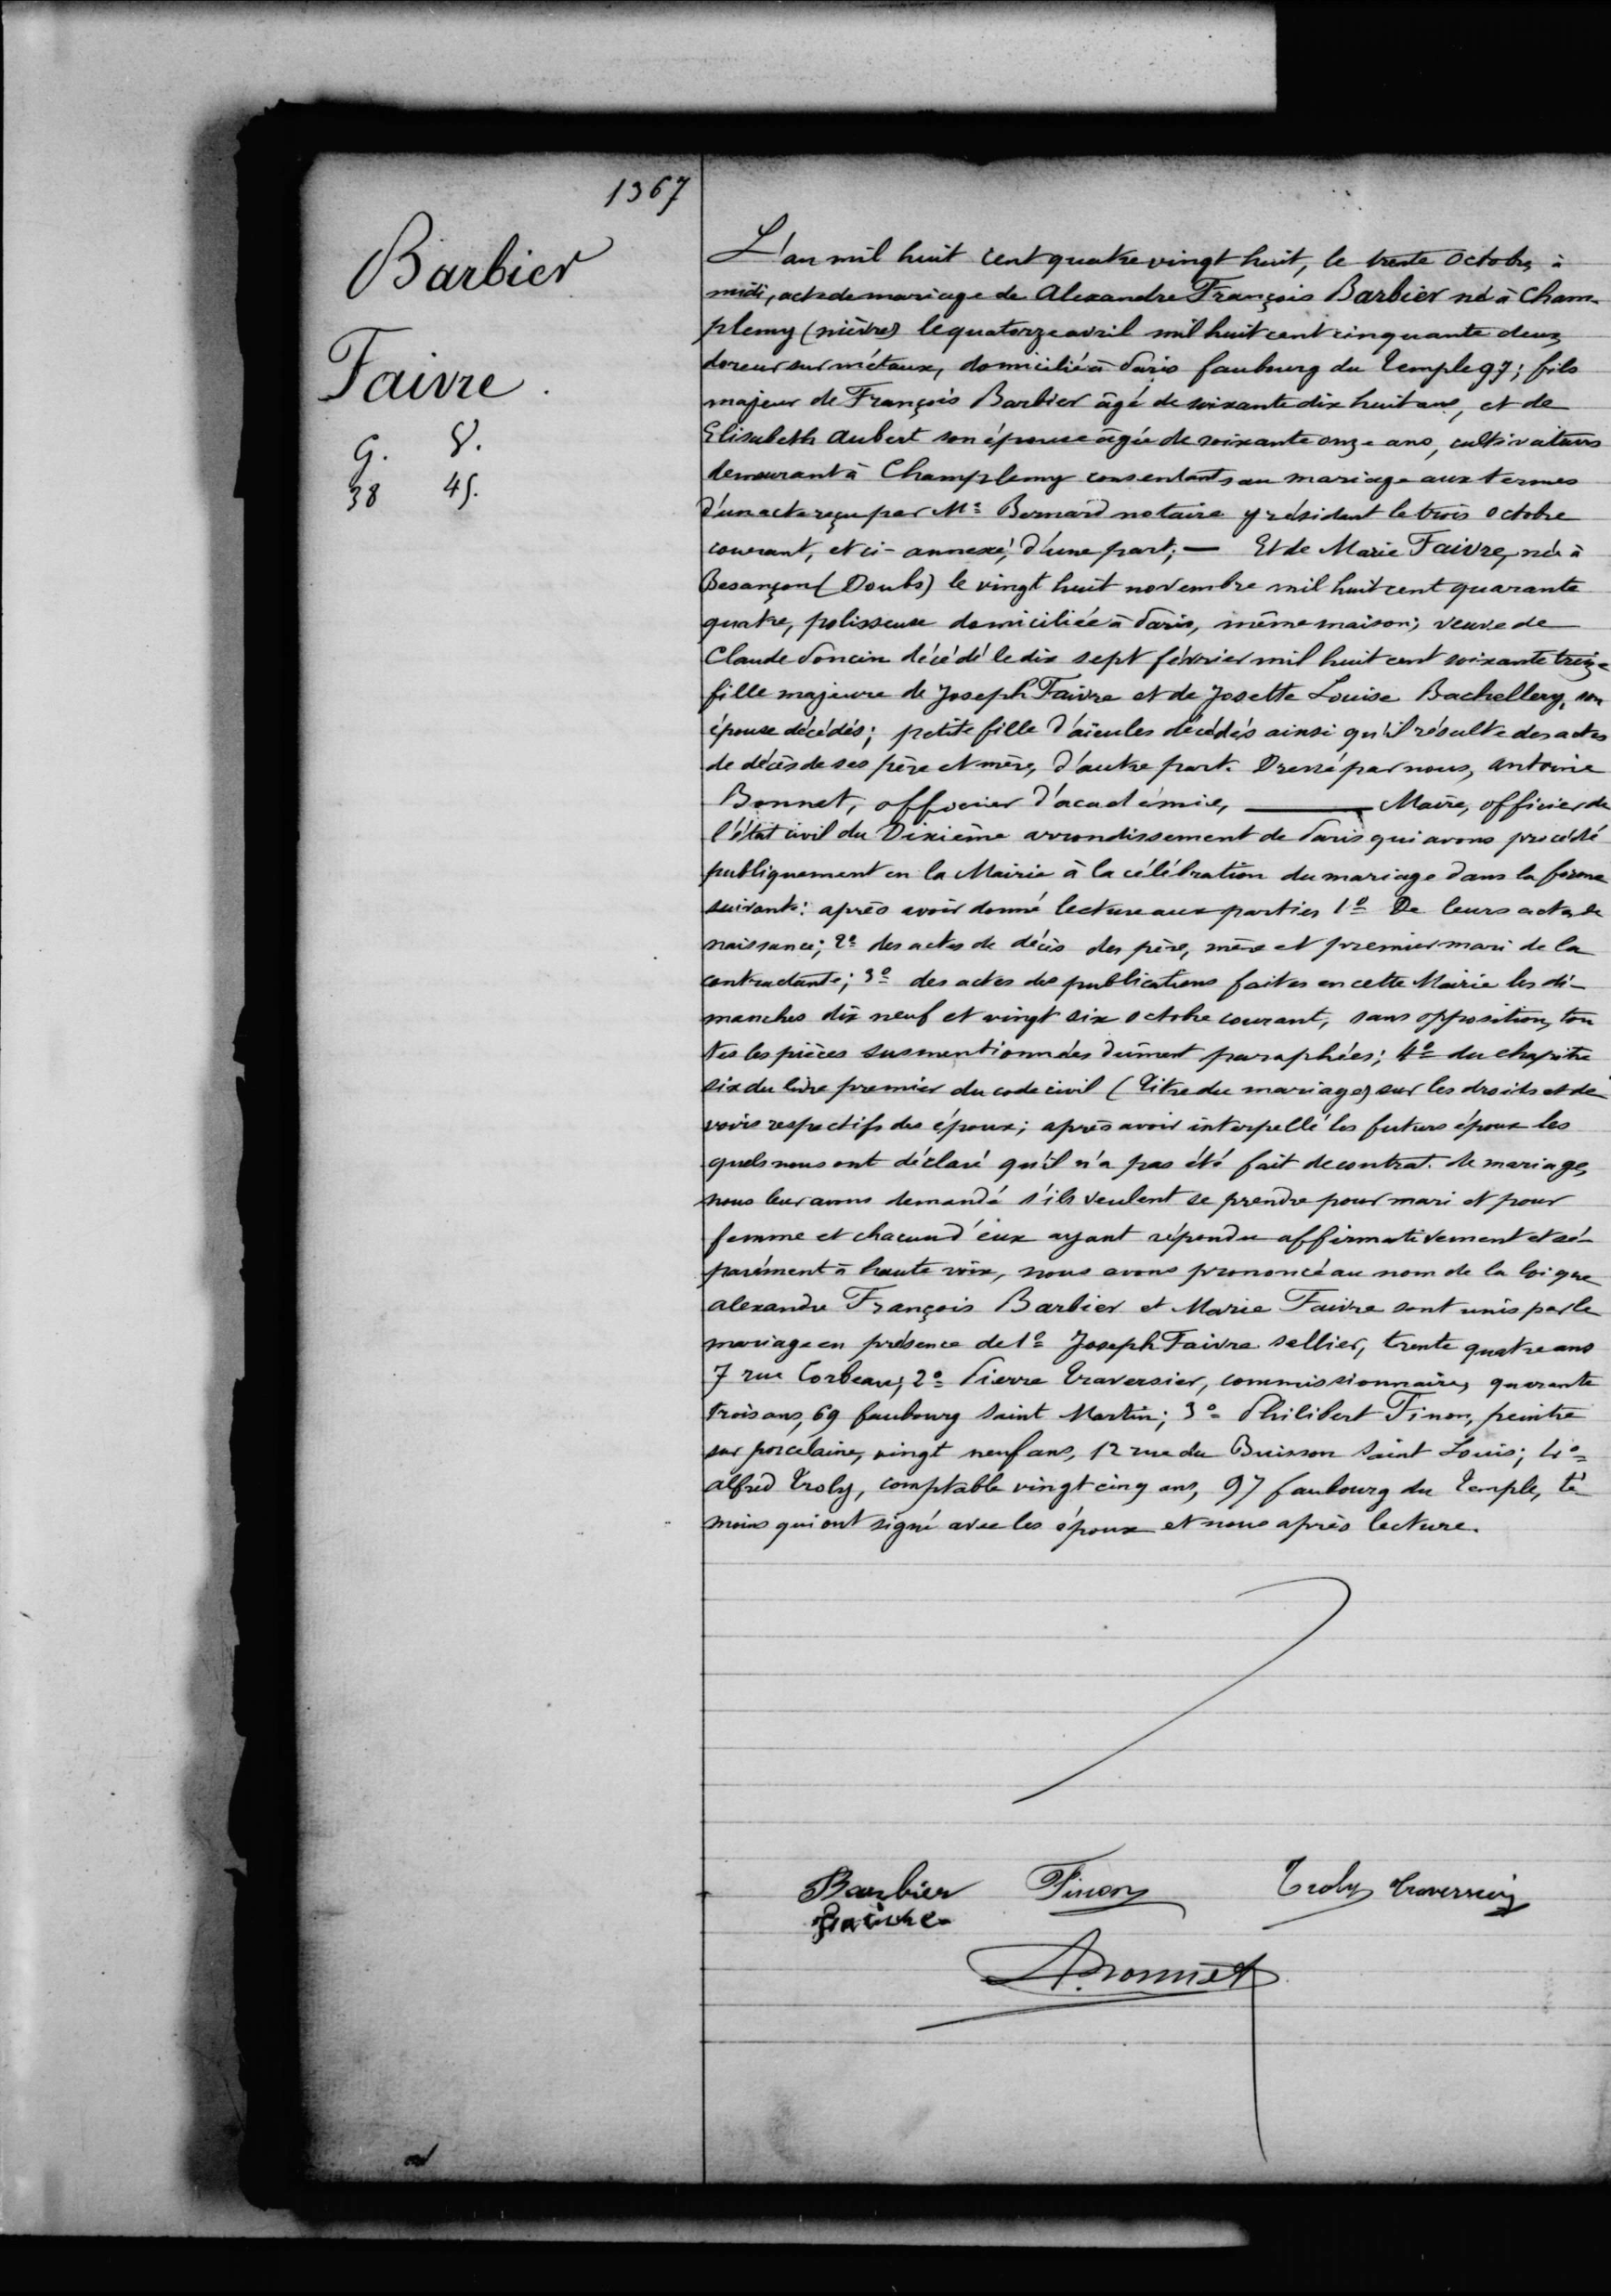1967
Barbier
Faivre
G. V.
38 45
L'an mil huit cent quatre vingt huit, le trente octobre à
midi, actedemariage de Alexandre François Barbier né à Cham-
plemy (Nièvres) le quatorze avril mil huit cent cinquante deux
doreur sur méteaux, domiciliés à Paris faubourg du Temple 97; fils
majeur de François Bartier âgé de soixante dix huit ans, et de
Elisabeth Aubert son épouse âgée de soixante onze ans, cultivateurs
demeurant à Complemy consentants au mariage aux terme
d'un acte reçu par M° Bosnard notaire préseidant le trois octobre
courant, et ci-annexé, d'une part; -Et de Marie Faivre, nè à
Besançon (Doubs) le vingt huit novembre mil huit cent quarante
quatre, polisseuse domiciliée à Paris, même maison; veuve de
Claude Soncine décédé le dix sept février mil huit cent soixante treize
fille majeure de Joseph Faivre et de Josette Louise Bachellery, son
épouse décédés; petite fille d'aïeules décédés ainsi qu'il résulte des actes
de décès de ses père et mère, d'autre part. Dressé par nous, antoine
Bonnet, officier d'académie, -Maire, officier de
l'état civil du Dixième arrondissement de Paris qui avons procédé
publiquement en la Mairie à la célébration du mariage dans la forme
suivante: après avoir donné lecture aux parties 1° De leurs actes de
naissance; 2° des actes de décès du père, mère et premier mari de la
contractante; 3° des actes de publications faites en cette Mairie les di-
manches dix neuf et vingt six octobre courant, sans opposition, tou
tes les pièces susmentionnées dûment paraphées; 4° du chapitre
six du livre premier du code civil (Titre du mariage) sur les droits et de-
voirs respectifs des époux; après avoir interpellé les futurs époux les
quels nout ont déclaré qu'il n'a pas été fait de contrat de mariage,
nous leur avons demandé s'ils veulent se prendre pour mari et pour
femme et chacun d'eux ayant répondu affirmativement et sé-
parèment à haute voix, nous avons prononcé au nom de la loi que
alexandre François Barbier et Marie Faivre son unis par le
mariage en présence de 1° Joseph Faivre Sellier, trente quatre ans
7 rue Corbeau; 2° Fierre Traversier, commissionnaire, quarante
trois ans, 69 faubourg Saint Martin; 3° Philibert Finon, peintre
sur porcelain, vingt neuf ans, 12 rue du Buisson Saint Louis; 4°
Alfre Troly, comptable vingt cinq ans, 97 faubourg de Temple, té-
moins qui ont signé avec les époux et nous après lecture.
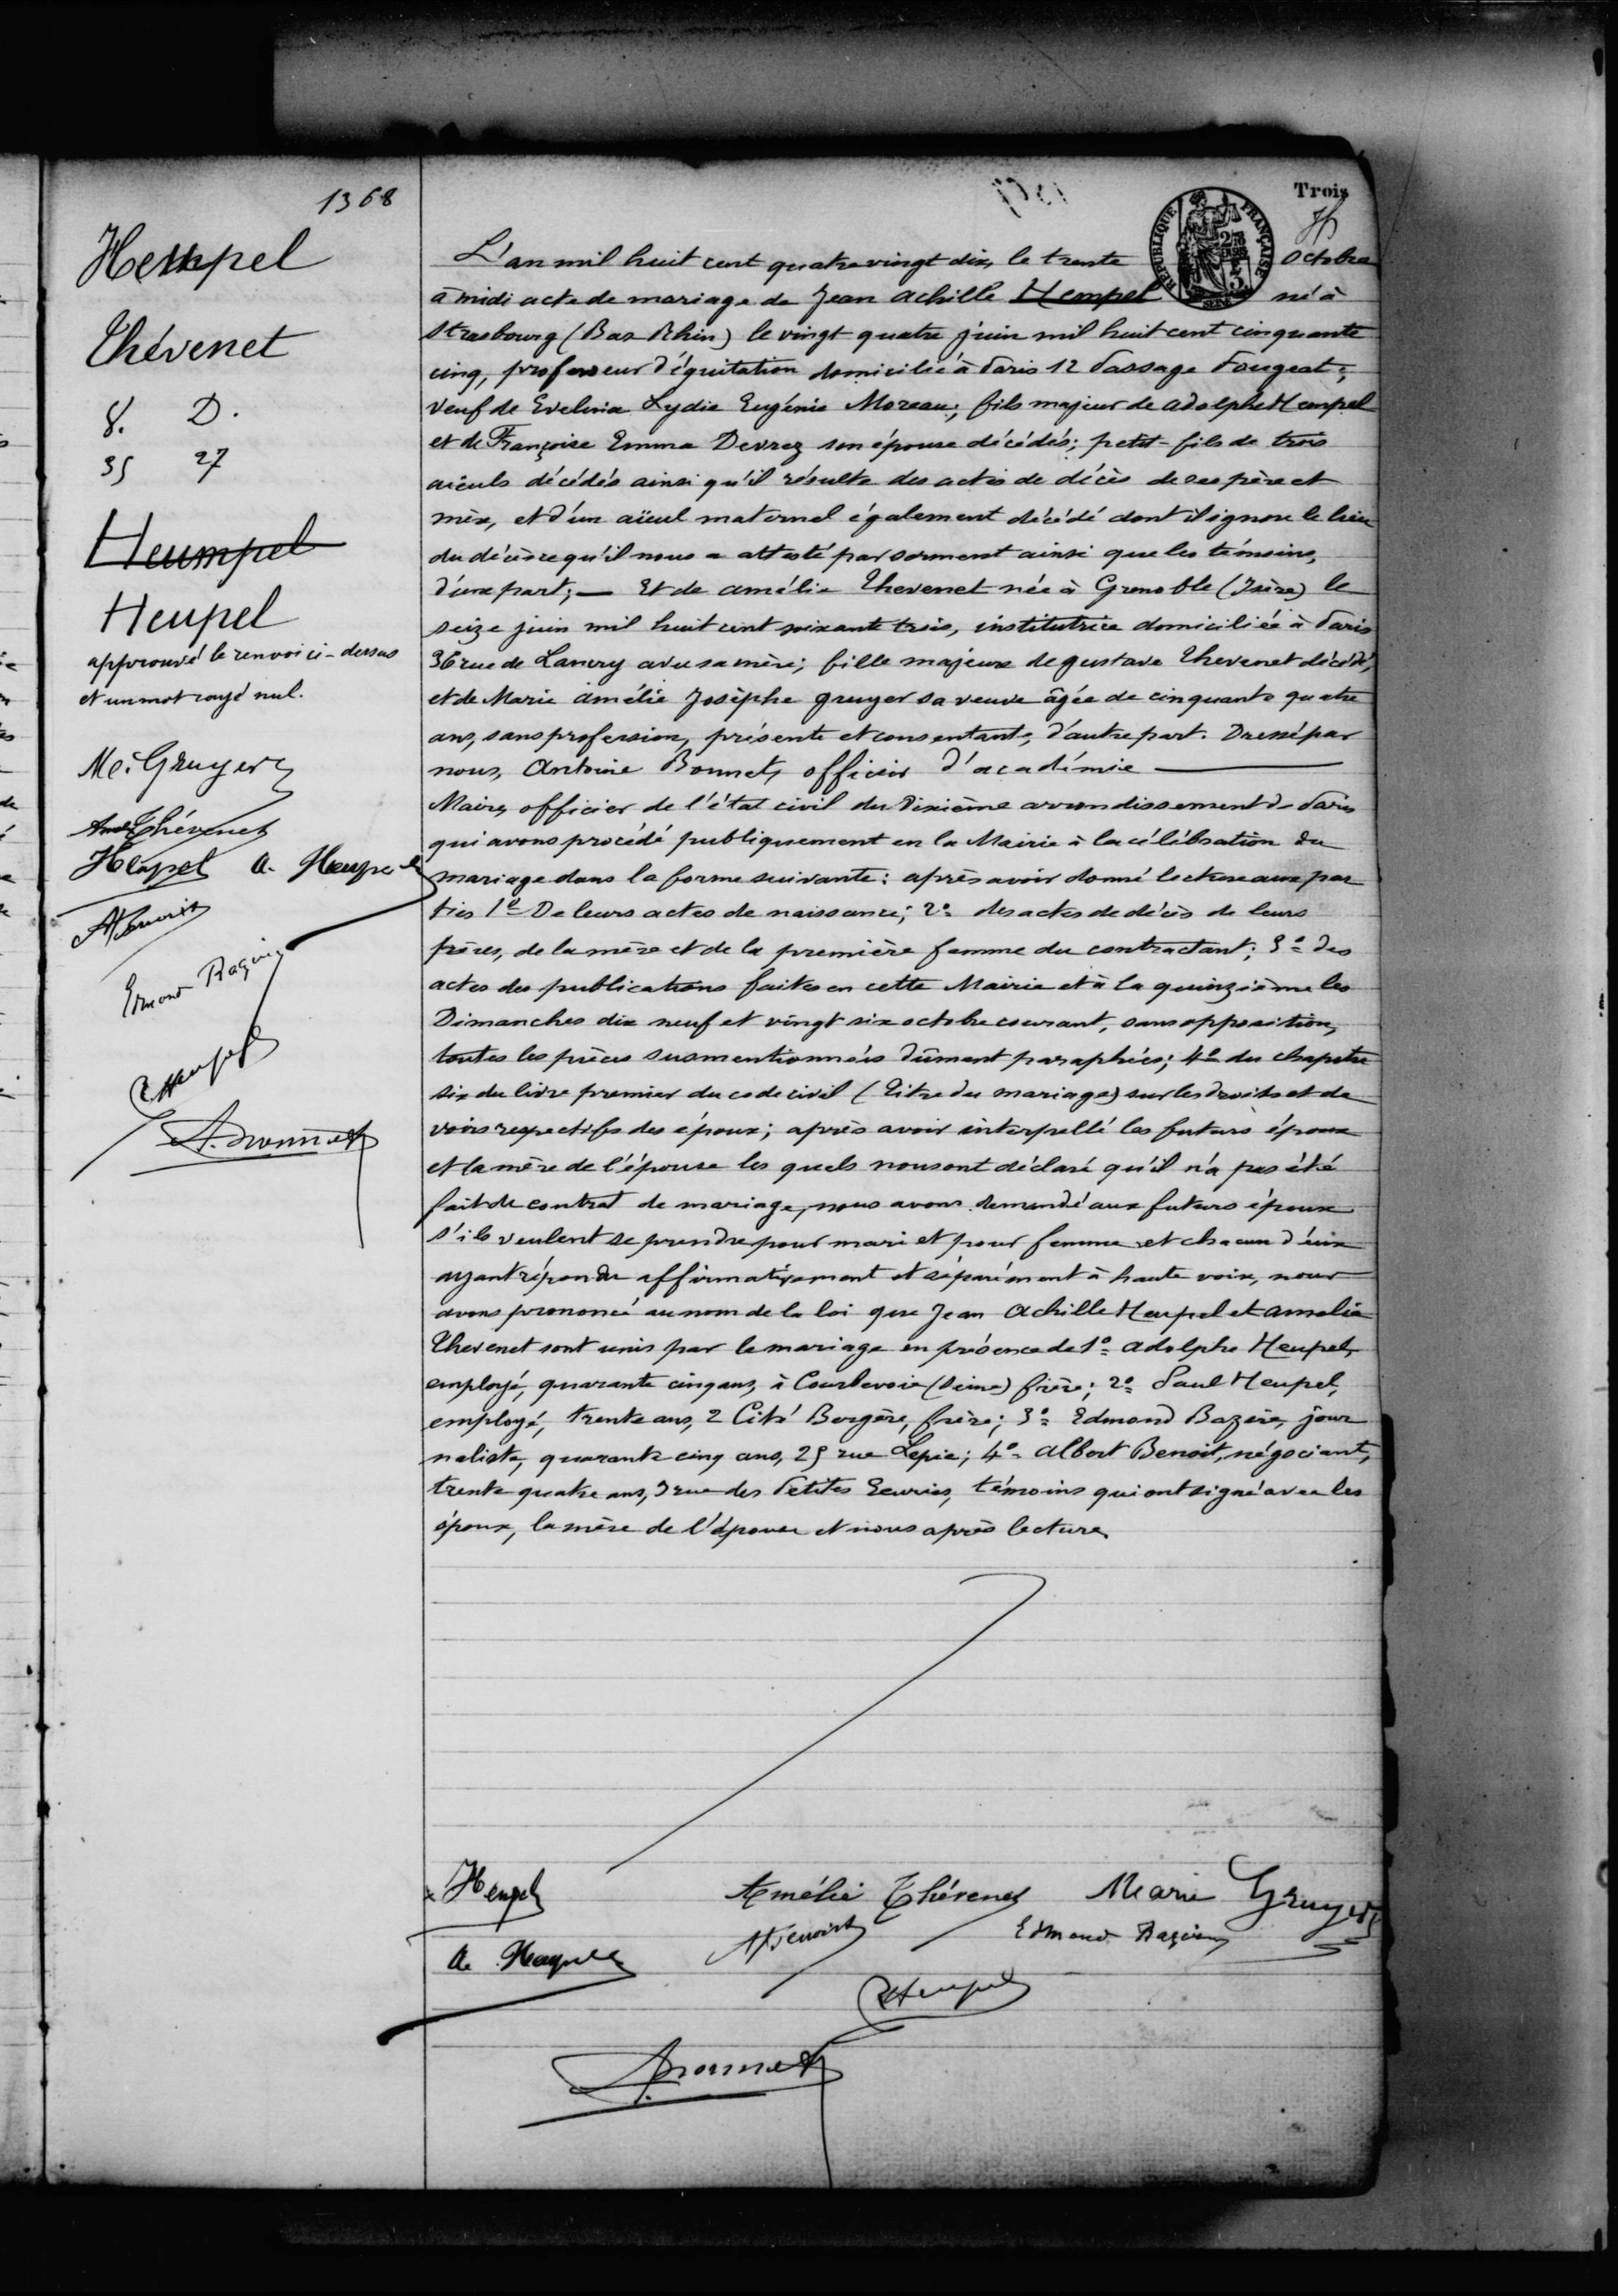1368
Hesspel
Chévenet
L'an mil huit cent quatre vingt dix, le trente octobre
à midi acte de mariage de Jean Achille Hempel né à
Strasbourg (Bas Rhin) le vingt quatre juin mil huit cent cinquante
cinq, professeur d'équitation domicilié à Paris 12 Passage Fougeat;
Veuf de Evelinia Lydia Eugénie Mozeau; fils majeur de adolphe Hempel
et de Françoise Emma Devrez son épouse décédés; petit-fils de trois
aieuls décédés ainsi qu'il résulte des actes de décès de ses père et
mère, et d'un aïeul maternel également décédé dont il ignore le lieu
du décès ce qu'il nous a attesté par serment ainsi que les témoins,
d'une part; -Et de amélia Thevenet née à Grenoble (Isère) le
seize juin mil huit cent soixante trois, institutrice domiciliée à Paris
96 rue de Lancry avec sa mère; fille majeure de Gustave Thevenet décédé,
et de Marie amélie Josèphe Gruyer sa veuve âgée de cinquante quatre
ans, sans profession, présent et consentant; d'autre part. Dressé par
nous, Antoine Bonnet, officier d'académie-
Maire, officier de l'état civil du dixième arrondissement, Paris
qui avons procédé publiquement en la Mairie à la célébration du
mariage, dans la forme suivante: après avoir donné lecture aux par
ties 1° De leurs actes de naissance; 2° des actes de décès de leurs
pères, de la mère et de la première femme du contractant; 3° des
actes des publications faites en cette Mairie et à la quinzième les
Dimanches dix neuf et vingt six octobre courant, sans opposition,
toutes les pièces susmentionnées dûment paraphées; 4° du chapitre
six du livre premier du code civil (Titre du mariage) sur les droits et de
voirs respectifs des époux; après avoir interpellé les futurs époux
et la mère de l'épouse les quels nous ont déclaré qu'il n'a pas été
fait de contrat de mariage, nous avons demandé aux futurs époux
s'ils veulent se prendre pour mari et pour femme et chacun d'eux
ayant répondu affirmativement et séparèment à haute voix, nous
avons prononcé au nom de la loi que Jean Achille Hempel et amelia
Chevenet sont unis par le mariage en présence de 1° Adolphe Henpel,
employé, quarante cinq ans, à Courbevoie (Seine) frère; 2° Paul Henpel
employé; trente ans, 2 Cité Bergère, frère; 3° Edmond Bazère jour
naliste, quarante-cinq ans, 25 rue Lepic; 4° Albert Benoit, négociant,
trente quatre ans, 9 rue des Petites Leuriers, témoins qui ont signé avec les
époux, la mère de l'épouse et nous après lecture.